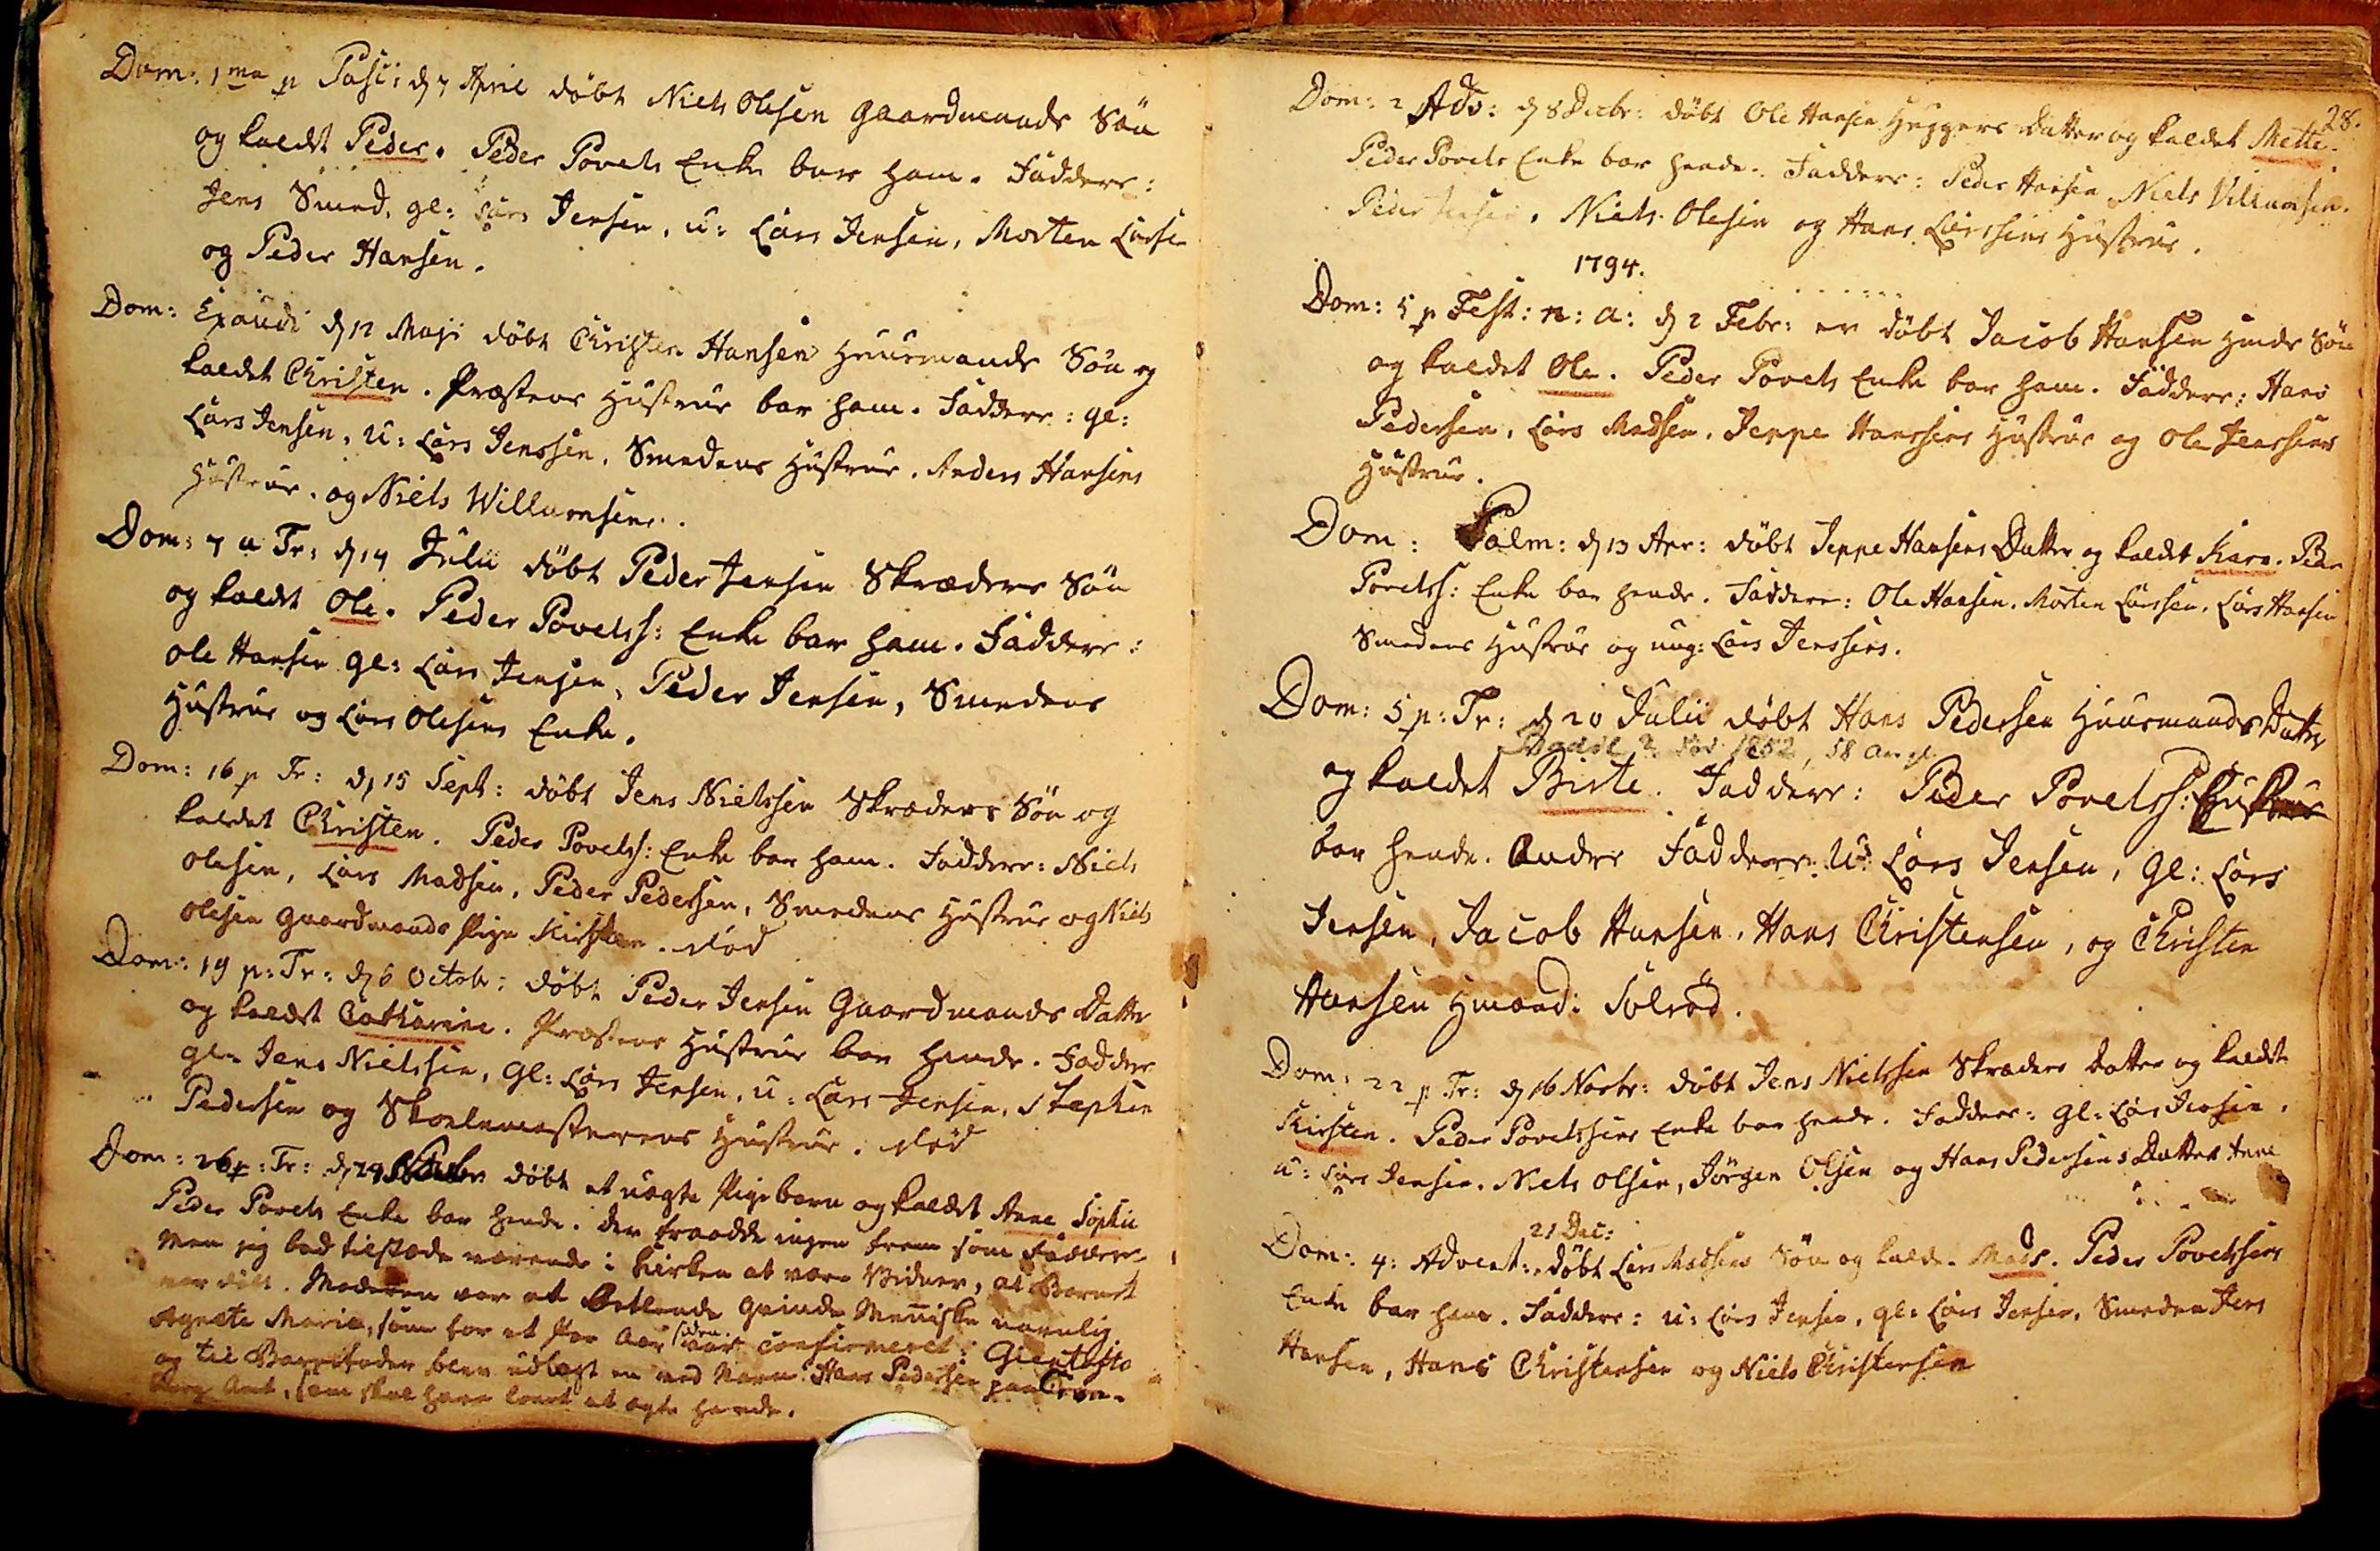Dom: 1ma p Pasc: d 7 April døbt Niels Olesen Gaardmands Søn
og kaldet Peder. Peder Povels Enke bar ham. Faddere:
Jens Smed, gl: Lars Jensen, u: Lars Jensen, Morten Larsen
og Peder Hansen.
Dom: Exaudi d 12 Maji døbt Christen Hansen Huusmands Søn og
kaldet Christen. Præstens Hustrue bar ham. Faddere: gl:
Lars Jensen, u: Lars Jenssen. Smedens Hustrue. Anders Hansens
Hustrue, og Niels Willumsens.
Dom: 7 e Tr: d 14 Juli døbt Peder Jensen Skræders Søn
og kaldet Ole. Peder Povelss: Enke bar ham. Faddere:
Ole Hansen Gl: Lars Jensen, Peder Jensen, Smedens
Hustrue og Lars Olesens Enke.
Dom: 16 p Tr: d 15 Sept: døbt Jens Nielsen Skræders Søn og
kaldet Christen. Peder Povelss: Enke bar ham. Faddere: Niels
Olesen, Lars Madsen, Peder Pedersen, Smedens Hustrue og Niels
Olesen Gaardmands Pige Kirsten. død
Dom: 19 p: Tr: d 6 Octobr: døbt Peder Jensen Gaardmands Datter
og kaldet Catharine. Præstens Hustrue bar hende. Faddere
gl: Jens Nielsen, gl: Lars Jensen, u: Lars Jensen, Stephen
Pedersen og Skolemesterens Hustrue. død
Dom: 26 p: Tr: d 24 Novbr: døbt et uægte Pigebarn og kaldet Anne Sophie
Peder Povels Enke bar hende. Der traadte ingen frem som Faddere
men jeg bad tilstædeværende i Kirken at være Vidner, at Barnet
var døbt. Moderen var et Betlende Qvinde Meniske navnlig
siden
Agnete Marie, som for et Par Aar var confirmeret i Gientofte
og til Barnefader blev udlagt en ved Navn Hans Pedersen paa Cron¬
borg Amt, som skal have lovet at ægte hende.
28.
Dom: 2 Adv: d 8 Decbr: døbt Ole Hansen Huggers Datter og kaldet Mette.
Peder Povels Enke bar hende. Faddere: Peder Hansen, Niels Villumsen,
Peder Jensen, Niels Olesen og Hans Larssens Hustrue.
1794.
Dom: 5 p Fest: n: a: d 2 Febr: er døbt Jacob Hansen Hmds Søn
og kaldet Ole. Peder Povels Enke bar ham. Faddere: Hans
Pedersen, Lars Madsen, Jeppe Hanssens Hustrue og Ole Jenssens
Hustrue.
Dom: Palm: d 13 Apr: døbt Jeppe Hansens Datter og kaldet Karn. Peder
Povelss: Enke bar hende. Faddere: Ole Hansen, Morten Larssen, Lars Hansen
Smedens Hustrue og ung: Lars Jenssens.
Dom: 5 p: Tr: d 20 Julii døbt Hans Pedersen Huusmands Datter
Bodil ? død 1852, 58 Aar gl.
og kaldet Birte. Faddere: Peder Povelss: Enke
bar hende. Andre Faddere: U: Lars Jensen, Gl: Lars
Jensen, Jacob Hansen, Hans Christensen, og Christen
Hansen Hmand i Solrød.
Dom: 22 p Tr: d 16 Novbr: døbt Jens Nielssen Skræders Datter og kaldet
Kirsten. Peder Povelssen Enke bar hende. Faddere: Gl: Lars Jensen,
u: Lars Jensen, Niels Olsen, Jørgen Olsen og Hans Pedersens Datter Anne
21 Dec:
Dom: 4: Advent: døbt Lars Madsens Søn og kaldet Mads. Peder Povelssens
Enke bar ham. Faddere: u: Lars Jensen, gl: Lars Jensen, Smeden Jens
Hansen, Hans Christensen og Niels Christensen
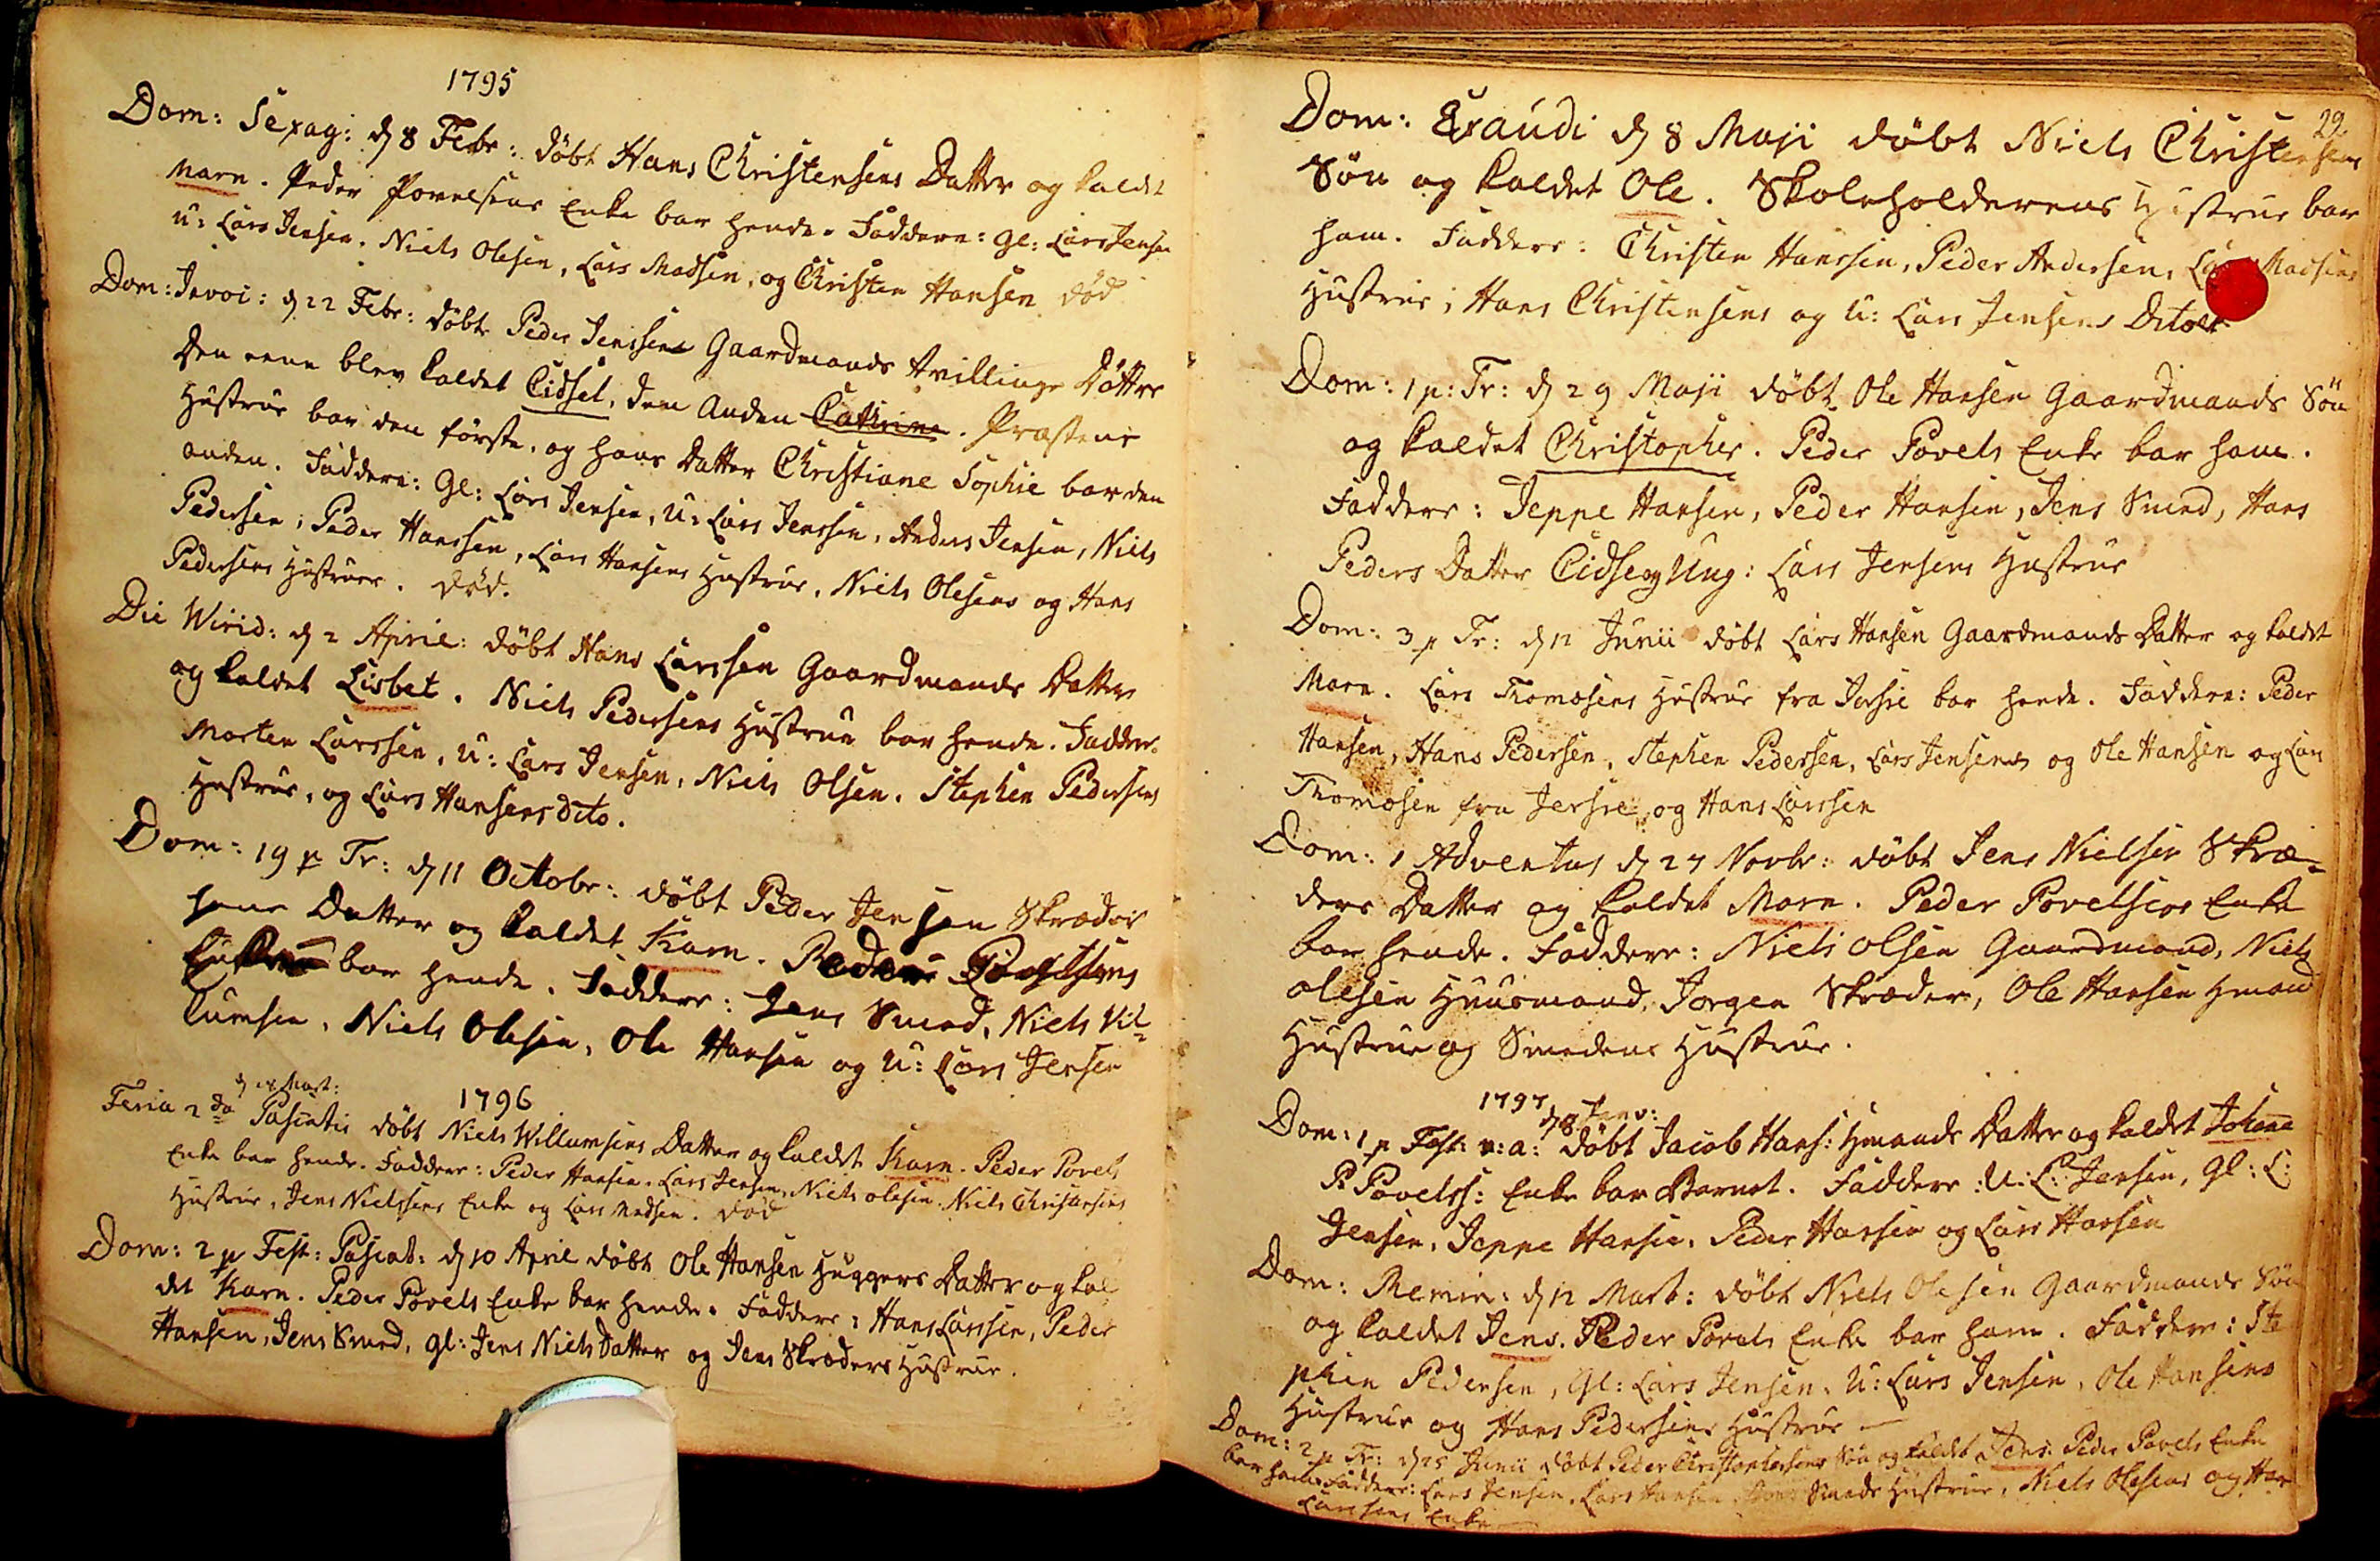1795
Dom: Sexag: d 8 Febr: døbt Hans Christensens Datter og kaldet
Marn. Peder Povelsens Enke bar hende. Faddere: Gl: Lars Jensen
u: Lars Jensen, Niels Olesen, Lars Madsen, og Christen Hansen. død
Dom: Invoc: d 22 Febr: døbt Peder Jenssen Gaardmands Tvillinge Døttre
Den eene blev kaldet Cidsel, den Anden Cathrine. Præstens
Hustrue bar den første, og hans Datter Christiane Sophie bar den
anden. Faddere: Gl: Lars Jensen, U: Lars Jenssen, Anders Jensen, Niels
Pedersen, Peder Hanssen, Lars Hansens Hustrue, Niels Olesens og Hans
Pedersens Hustruer. død.
Die Wirid: d 2 April: døbt Hans Larssen Gaardmands Datter
og kaldet Lisbet. Niels Pedersens Hustrue bar hende. Faddere:
Morten Larssen, u: Lars Jensen, Niels Olsen, Stephen Pedersens
Hustrue, og Lars Hansens dito.
Dom: 19 p Tr: d 11 Octobr: døbt Peder Jensen Skræder
hans Datter og kaldet Karn. Peder Povelsens
Enke bar hende. Faddere: Jens Smed, Niels Vil¬
lumsen, Niels Olesen, Ole Hansen og u: Lars Jensen
1796
d 28 Mart:
Feria 2da Pascatis døbt Niels Willumsens Datter og kaldet Karn. Peder Povels
Enke bar hende. Faddere: Peder Hansen, Lars Jensen, Niels Olesen, Niels Christensens
Hustrue, Jens Nielsens Enke og Lars Madsen. død
Dom: 2 p Fest: Pascat: d 10 April døbt Ole Hansen Huggers Datter og kal
det Karn. Peder Povels Enke bar hende. Faddere: Hans Larssen, Peder
Hansen, Jens Smed, gl: Jens Niels Datter og Jens Skræders Hustrue.
29.
Dom: Exaudi d 8 Maji døbt Niels Christensens
Søn og kaldet Ole. Skoleholderens Hustrue bar
ham. Faddere: Christen Hanssen, Peder Andersen, Lars Madsens
Hustrue, Hans Christensens og U: Lars Jensens Ditoe
Dom: 1 p: Tr: d 29 Maji døbt Ole Hansen Gaardmands Søn
og kaldet Christopher. Peder Povels Enke bar ham.
Faddere: Jeppe Hansen, Peder Hansen, Jens Smed, Hans
Peders Datter Cidse og Ung: Lars Jensens Hustrue
Dom: 3 p Tr: d 12 Junii døbt Lars Hansen Gaardmands Datter og kaldet
Marn. Lars Thomæsens Hustrue fra Jersie bar hende. Faddere: Peder
Hansen, Hans Pedersen, Stephen Pedersen, Lars Jensen og Ole Hansen og Lars
Thomæsen fra Jersie og Hans Larssen
Dom: 1 Adventus d 27 Novbr: døbt Jens Nielsen Skræ¬
ders Datter og kaldet Marn. Peder Povelsens Enke
bar hende. Faddere: Niels Olsen Gaardmand, Niels
Olesen Huusmand, Jørgen Skræder, Ole Hansen Hmand
Hustrue og Smedens Hustrue.
1797
d 8 Janu:
Dom: 1 p Fest: n: a: døbt Jacob Hans: Hmands Datter og kaldet Johane.
P: Povelss: Enke bar Barnet. Faddere: U: L: Jensen, gl: L:
Jensen, Jeppe Hansen, Peder Hansen og Lars Hensen
Dom: Remin: d 12 Mart: døbt Niels Olesen Gaardmands Søn
og kaldet Jens. Peder Povels Enke bar ham. Faddere: Ste
phen Pedersen, Gl: Lars Jensen, U: Lars Jensen, Ole Hansens
Hustrue og Hans Pedersens Hustrue
Dom: 2 p Tr: d 25 Junii er døbt Peder Christophersens Søn og kaldet Jens. Peder Povels Enke
bar ham. Faddere: Lars Jensen, Lars Hansen, Jens Smeds Hustrue, Niels Olesens og Hans
Larsens Enke.